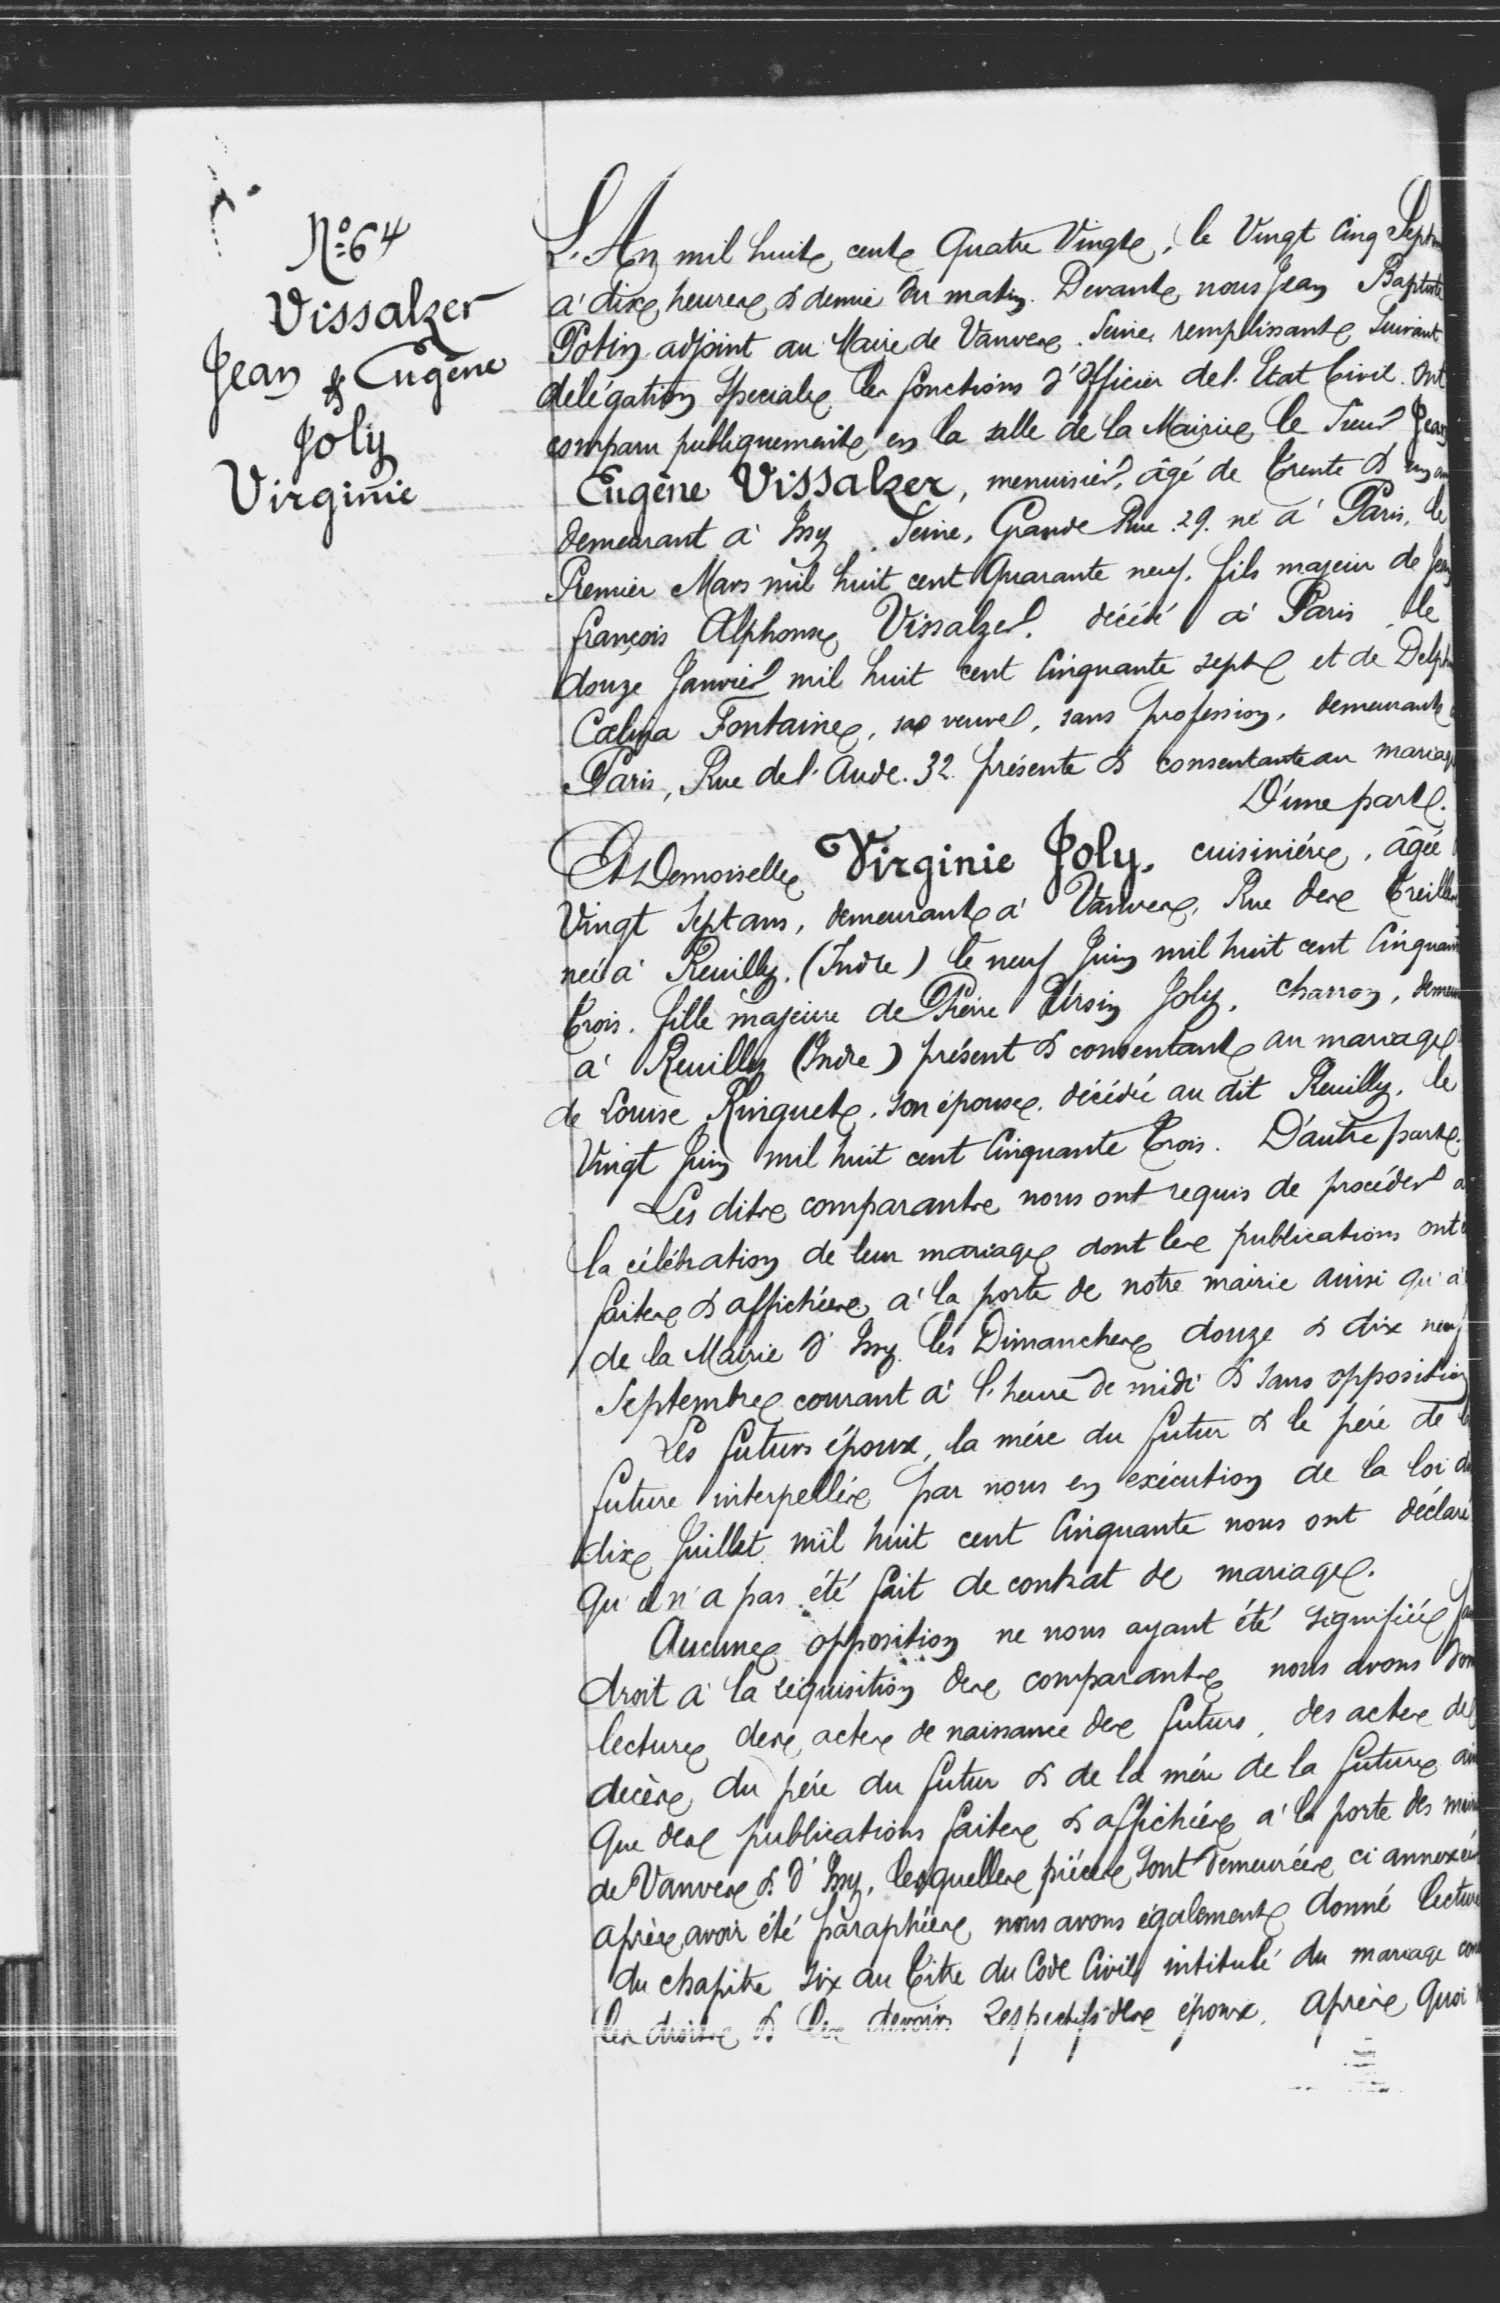N°64
Vissalzer
Jean & Eugène
Joly
Virginie
L'An mil huit cent Quatre Vingt, le Vingt Cinq Sept
à dix heures et demie du matin. Devant nous Jean Baptiste
Potin adjoint au Maire de Vanvre Seine remplissant Suivant
délégation Speciale les fonctions d'Officier de l'Etat Civil. Ont
comparu publiquement en la salle de la Mairie le Sieur Jean
Eugène Vissalzer, menuisier, âgé de Trente et un an
demeurant à Issy Seine, Grande Rue 29 né à Paris, le
Premier Mars mil huit cent Quarante neuf, fils majour de Jean
François Alphonse Vissalzer décédé à Paris le
douze Janvier mil huit cent Cinquante sept et de Delph
Calina Fontaine, sa veuve, sans profession, demeurant à
Paris, Rue de l'Aube 32 présente et consentante au mariage
D'une part.
Demoiselle Virginie Joly, cuisinière, âgée
Vingt Sept ans, demeurant à Vanvre, Rue des Treille
née à Reuilly (Indre) le neuf Juin mil huit cent Cinquan
trois. fille majeure de Pierre Ursin Joly, charron dem
à Reuilly (Indre) présent et consentante au mariage
de Louise Ringuete, son épouse décédée au dit Reuilly, le
Vingt Juin mil huit cent Cinquante Trois. D'autre part.
Les dits comparants nous ont requis de procéder a
la célébration de leur mariage dont les publications ont é
faites d'affichées à la porte de notre mairie ainsi qu'à
de la Mairie d'Issy les Dimanches douze et dix neuf
Septembre courant à l'heure de midi & sans opposition.
Les futurs époux, la mère du futur & le père de la
future interpellée par nous en exécution de la loi du
dix Juillet mil huit cent Cinquante nous ont déclaré
Qu'il n'a pas été fait de contrat de mariage.
Aucune opposition ne nous ayant été signifiée Ja
droit à la réquisition des comparants nous avons do
lecture des actes de naissance des futurs, des actes de
décès du père du futur & et de la mère de la future ai
Que de la publication faite & affichée à la parte des mair
de Vanre & d'Issy, lesquelles pièces sont demeurées ci annexées
après avoir été paraphées nous avons également donné lecture
du chapitre six au Titre du Code Civil intitulé du mariage nou
les droits & les devoirs respectifs des époux après Quoi
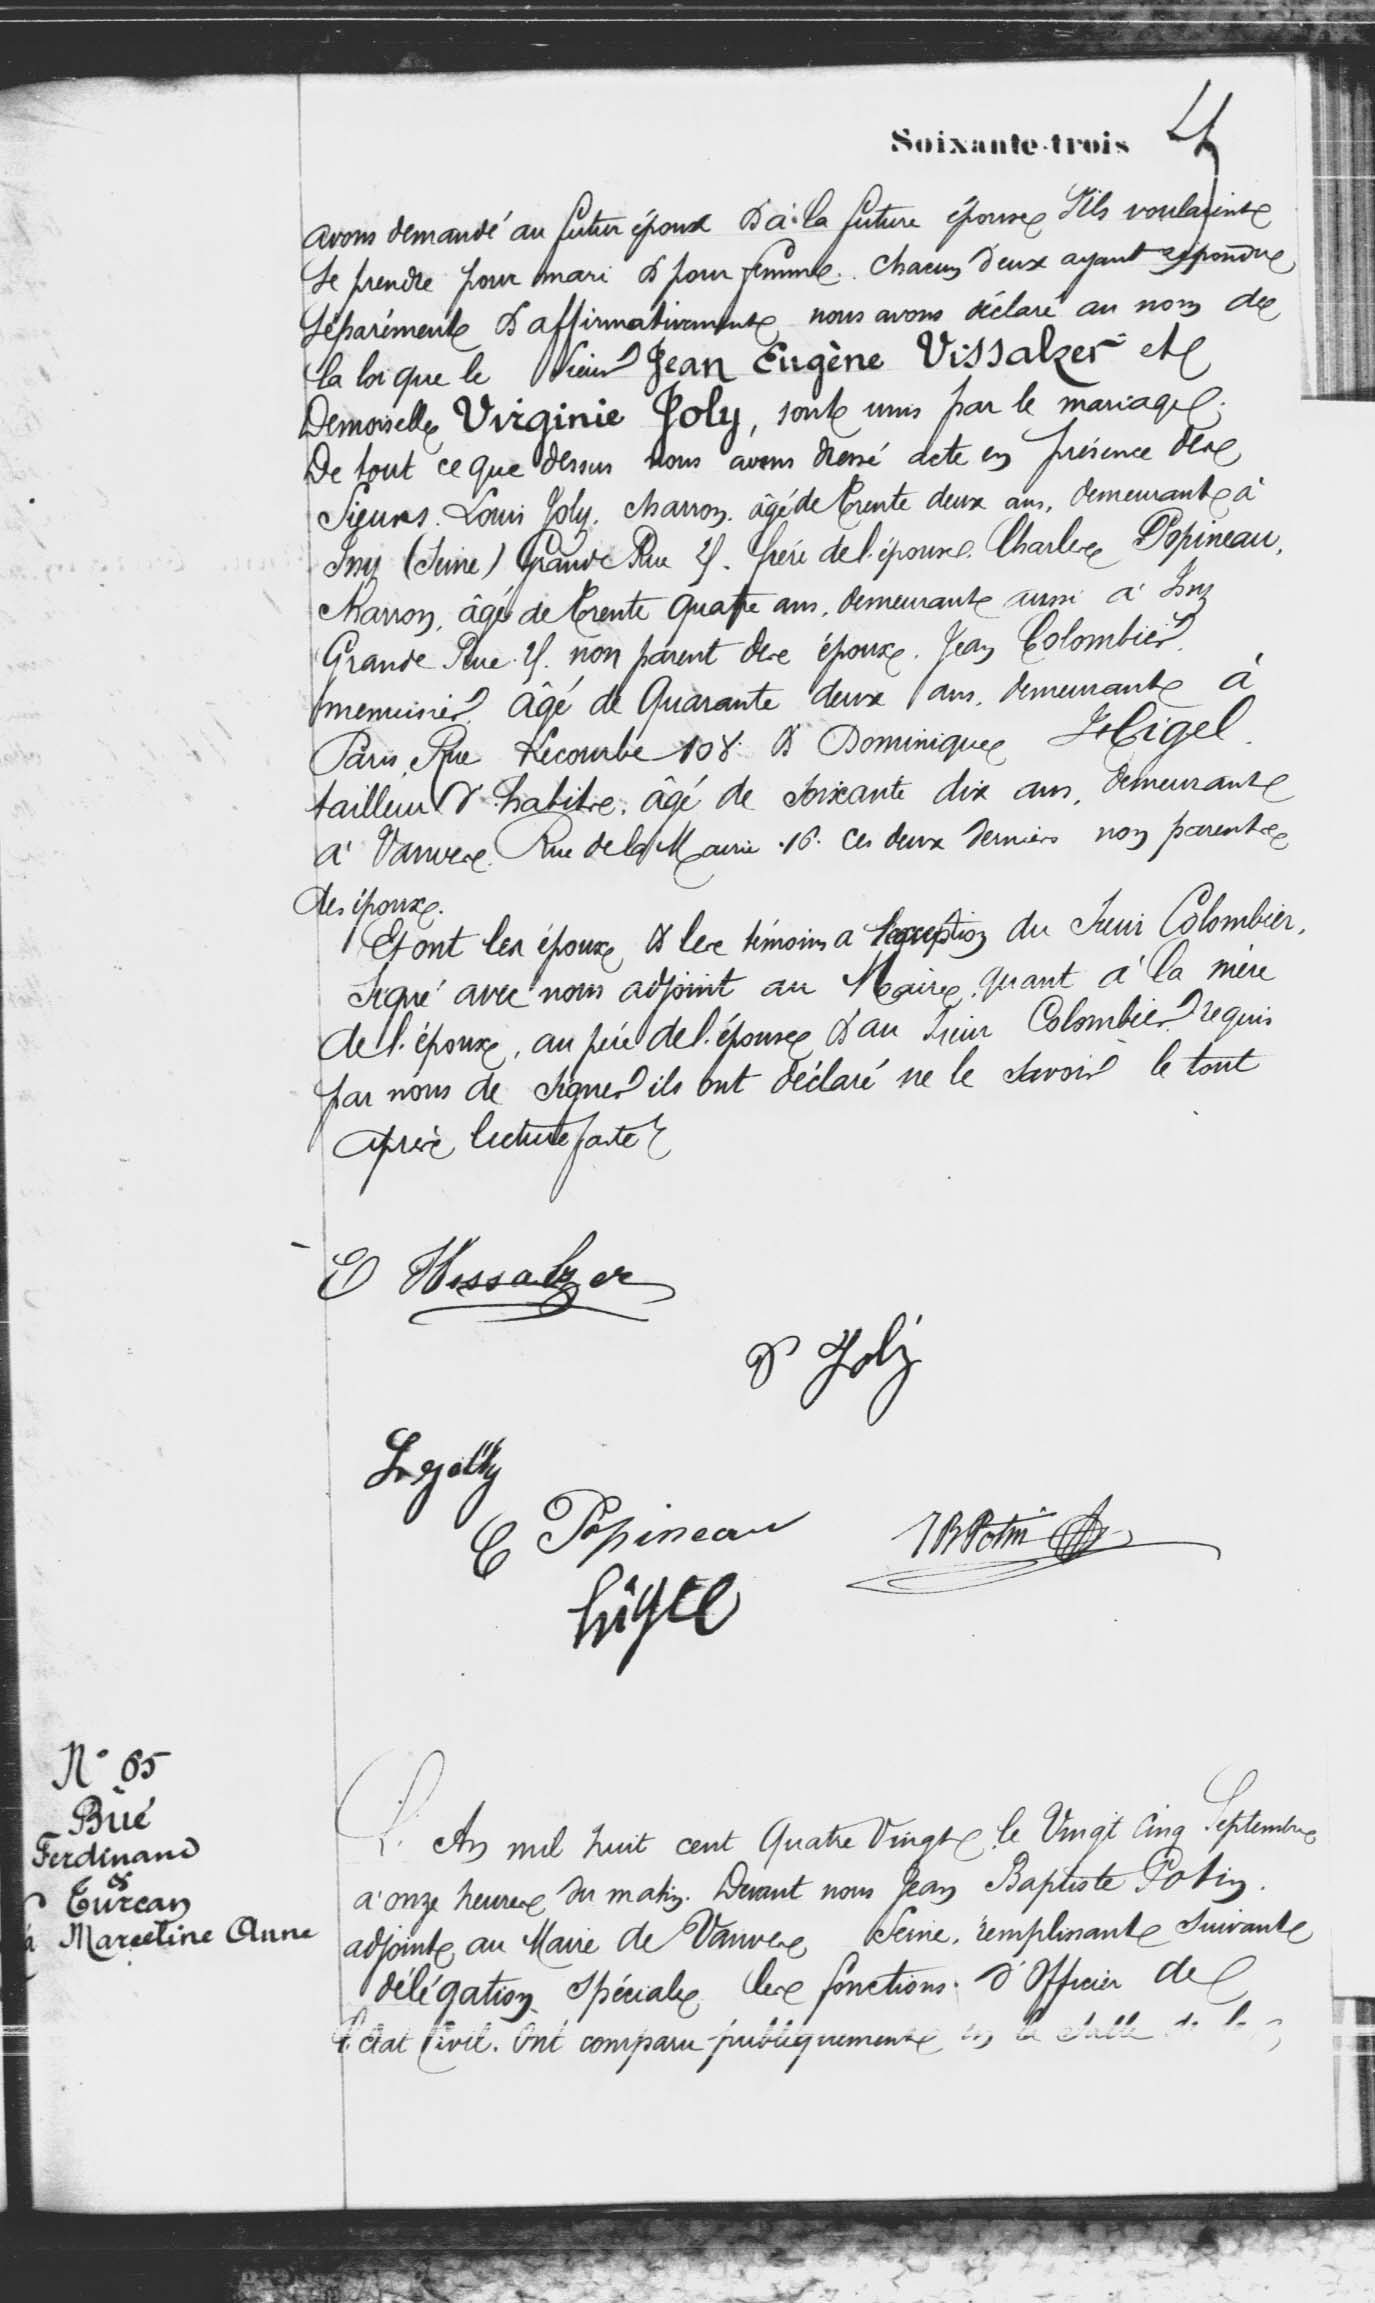Avons demandé au futur époux & à la future épouse S'ils voulaient
Se prendre pour mari & pour femme. Chacun d'eux ayant approuve
Séparément & affirmativement nous avons déclaré au nom de
La loi que Sieur Jean Eugène Vissalzer et
Demoiselle Virginie Joly, sont unis par le mariage.
De tout ce que dessus nous avons donné acte en présence des
Sieurs Louis Joly charron âgé de trente deux ans, demeurant à
Issy (Seine) Grande Rue 29 frère de l'époux & Charles Popineau,
charron, âgé de trente quatre ans, demeurant aussi à Issy
Grande Rue 29 non parent de l'épouse, Jean Colombier,
menuisier âgé de Quarante deux ans demeurant à
Paris Rue Lecourbe 108 & Dominique Jiligel,
tailleur & habile, âgé de soixante dix ans, demeurant
à Vanvre Rue de la Mairie 16 ces deux derniers non parents
des époux.
Et ont les époux & les témoins à l'exception du Sieur Colombier
Signé avec nous adjoint au Mairie, Quant à la mère
de l'époux, au père de l'épouse & au Sieur Colombier requis
par nous de signer ils ont déclaré ne le savoir le tout
Après lecture faite
N°65
Bué
Ferdinand
&
Curcan
Marceline Anne
L'An mil huit cent Quatre Vingt le Vingt Cinq Septembre
à onze heures du matin. Devant nous Jean Baptiste Potin
adjointe au Maire De Vanves Seine, remplissant suivant
délégation spéciale les fonctions d'Officier de l
l'Etat Civil. Ont comparu publiquement en la salle de la